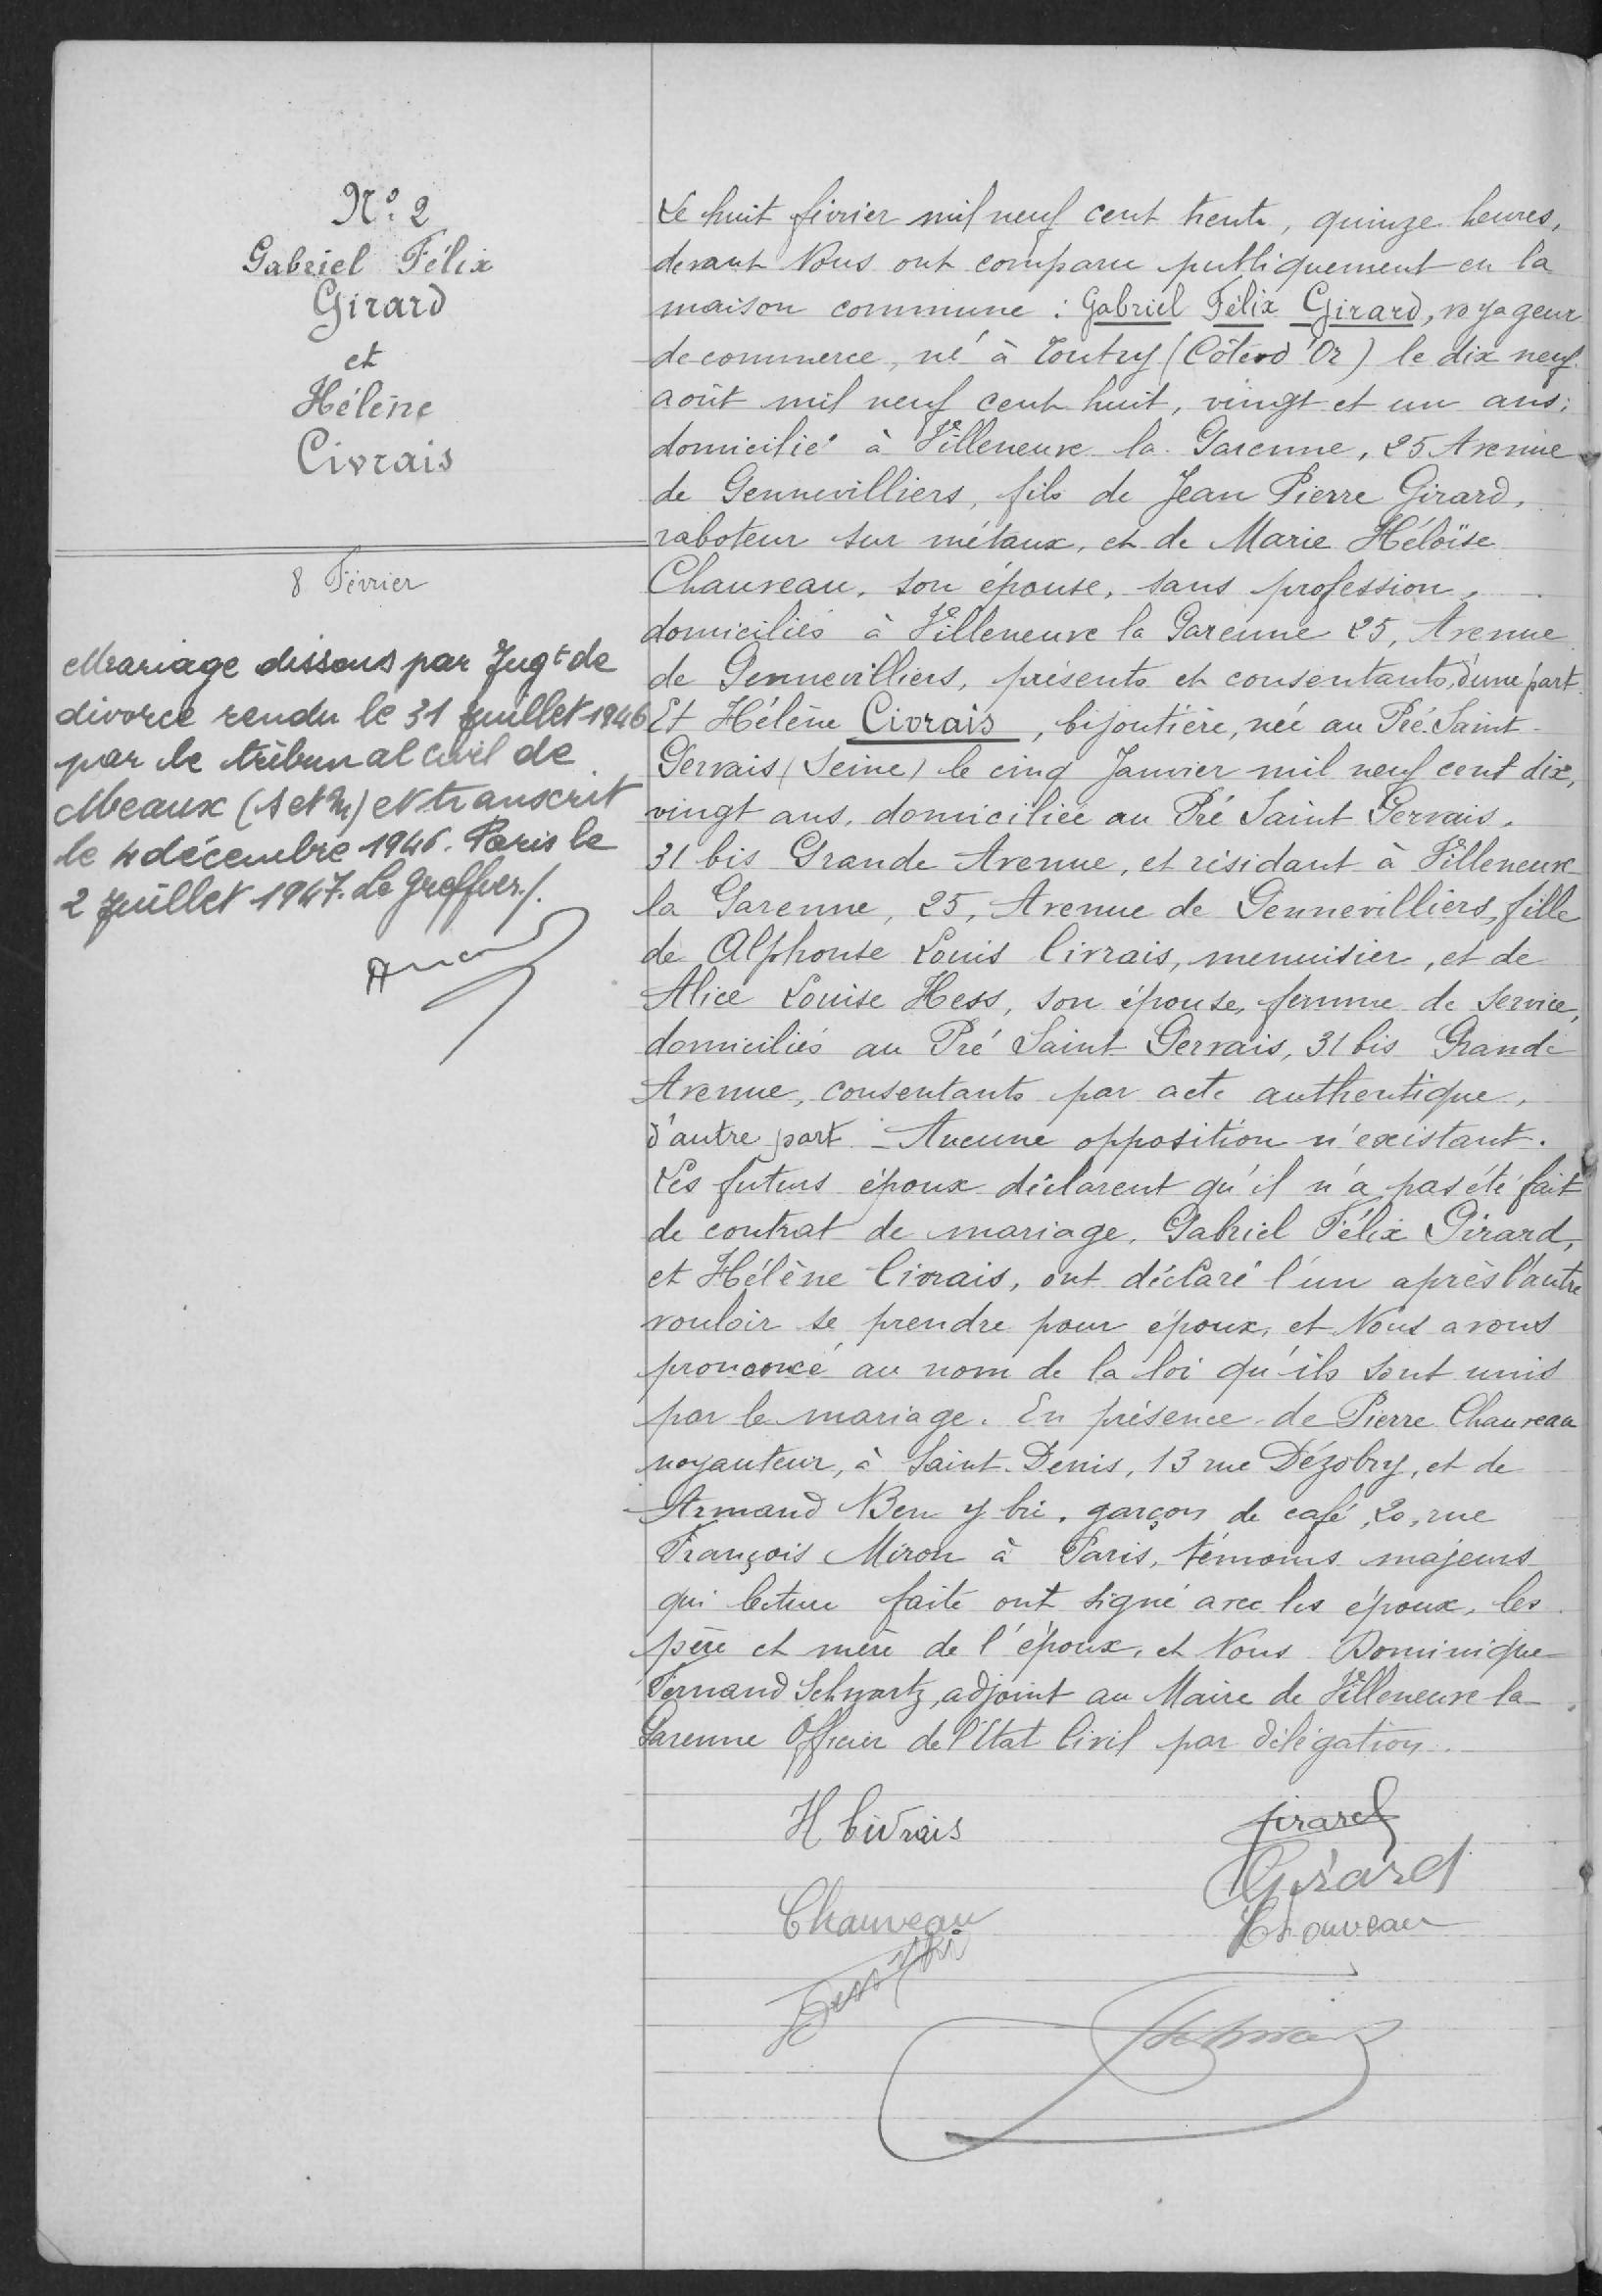N°2
Gabriel Félix
Girard
et
Hélène
Civrais
Le huit février mil neuf cent trente, quinze heures,
devant Nous ont comparu publiquement en la
maison commune: Gabriel Félix Girard, voyageur
de commerce, né à Toutry (Côte-d'Or) le dix neuf
août mil neuf cent huit, vingt et un ans;
domicilié à Villeneuve la Garenne, 25 Avenue
de Gennevilliers, fils de Jean Pierre Girard,
raboteur sur métaux, et de Marie Héloïse
Chauveau, son épouse, sans profession,
domiciliés à Villeuneuve la Garenne 25, Avenue
de Gennevilliers, présents et consentant, d'une part.
Et Hélène Civrais, bijoutières, née à Pré-Saint
Gévrais (Seine) le cinq Janvier mil neuf cent dix,
vingt ans, domiciliée au Pré Saint Gervais.
31 bis Grande Avenue, et résidant à Villeneuve
la Garenne, 25, Avenue de Gennevilliers, fille
de Alphonse Louis Civrais, menuisier, et de
Alice Louise Hess, son épousé femme de service
domiciliés au Pré Saint Gervais, 31 bis Grande
Avenue, consentants par acte authentique
d'autre part. Aucune opposition n'existant
Les futurs époux déclarent qu'il n'a pas été fait
de contrat de mariage. Gabriel Félix Gérard,
et Hélène Civrais, ont déclaré l'un après l'autre
vouloir se prendre pour époux et Nous avons
prononcé au nom de la loi qu'ils sont unis
par le mariage. En présence de Pierre Chaureau
noyauteur, à Saint-Denis, 13 rue Dézoby, et de
Armand Ben Y bi, garçon de café, 20, rue
François Miron à Paris, témoins majeurs
qui lecture faite ont signé avec les époux, les
père et mère de l'épouse et Nous Dominique
Fernand Schwartz, adjoint au maire Villeneuve la
Garenne Officier de l'Etat Civil par délégation
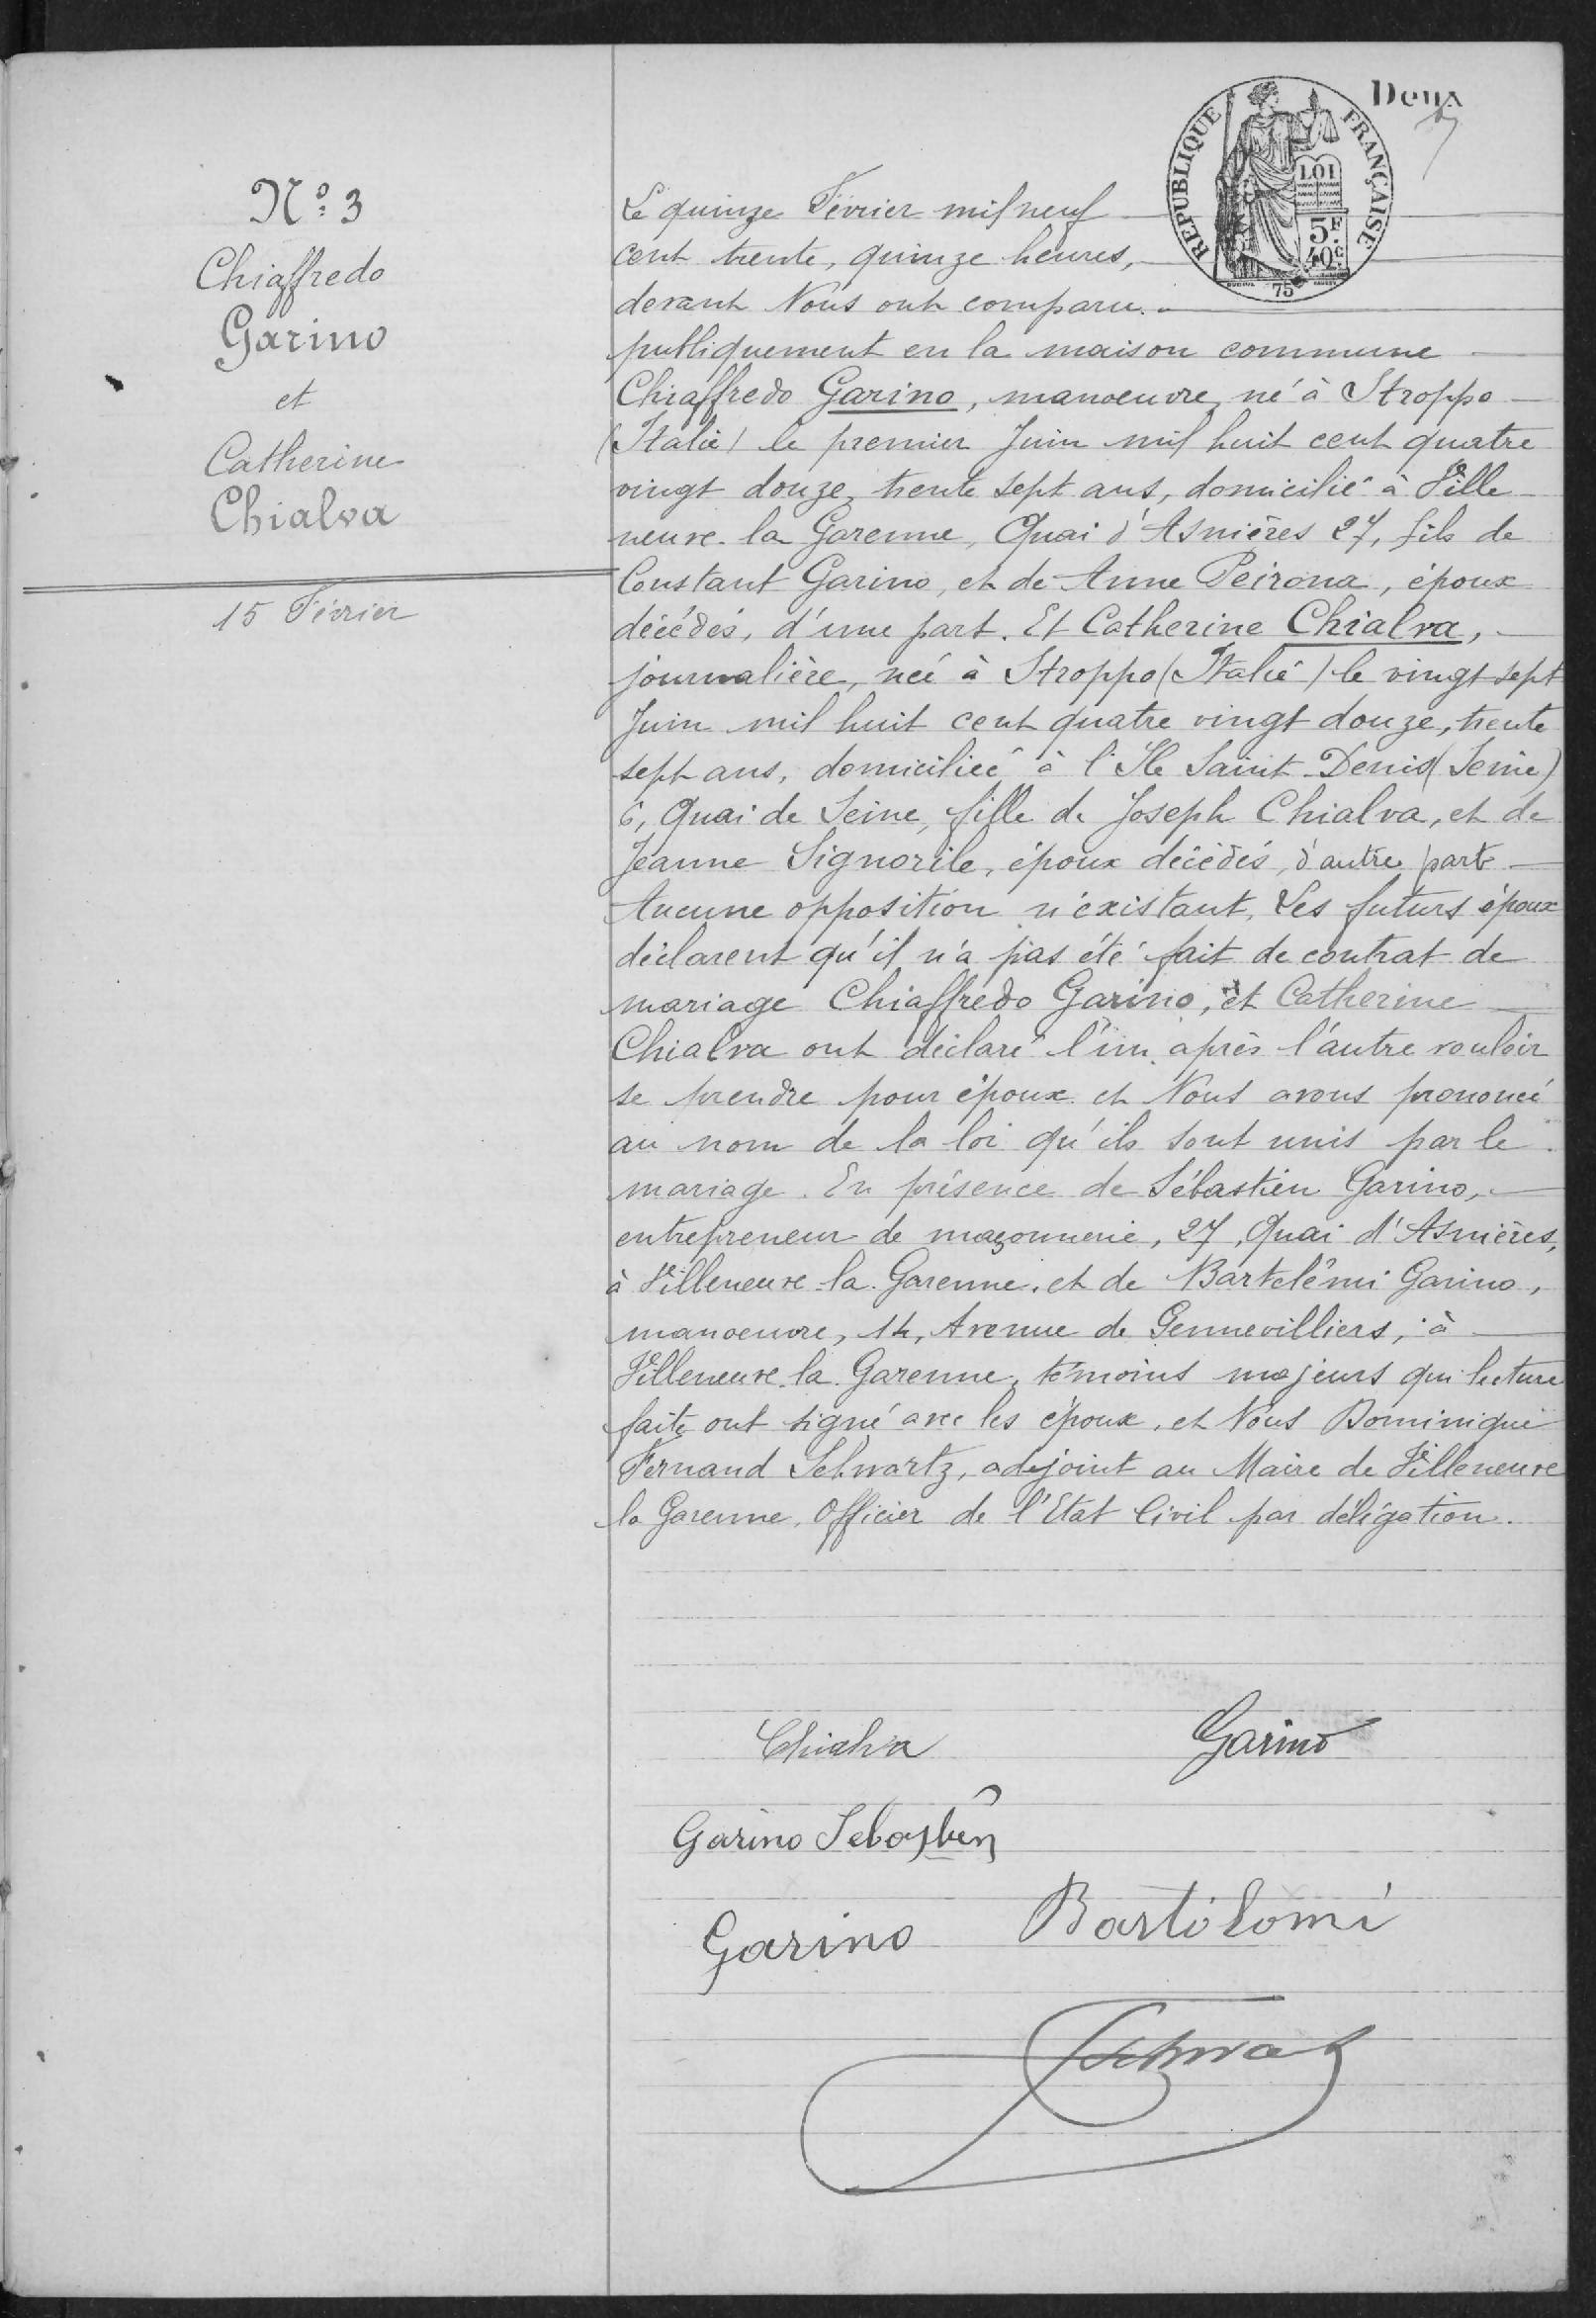N°3
Chiaffredo
Garino
et
Catherine
Chialva
15 février
Le quinze février mil neuf -
cent trente, quinze heures, -
devant Nous ont comparu -
publiquement en la maison commune -
Chiaffrdo Gorino, manoeuvre, né à Stroppo -
(Italie) le premier Juin mil huit cent quatre
vingt douze trente sept ans, domicilié à Ville
neuve la Garenne, Quai d'Asnières 27, fils de
Constant Garino, et de Anne Peirona, époux
décédés, d'une part. Et Catherine Chialva, -
journalière, née à Stroppo (Italie) le vingt-sept
Juin mil huit cent quatre vingt-douze, trente
sept ans, domiciliée à l'Ile Seint-Denis (Seine)
6, Quai de Seine, fille de Joseph Chialva, et de
Jeanne Signorile, époux décédés, d'autre part -
Aucune opposition n'existant, Les futurs époux
déclarent qu'il n'a pas été fait de contrat de
mariage Chiaffredo Garino, et Catherine -
Chialva ont déclaré l'un après l'autre vouloir
se prendre pour époux et Nous avons prononcé
au nom de la loi qu'ils sont unis par le
mariage. En présence de Sébastien Garino, -
entrepreneur de maçonnerie, 27, Quai d'Asnières,
à Villeneuve-la-Garenne, et de Bartelèmi Garino,
manoeuvre, 14, Avenue de Gennevilliers, à -
Villeneuve-la-Garenne, témoins majeurs qui lecture
faite ont signé avec les époux. et Nous Dominique
Fernand Schwartz, adjoint au Maire de Villeneuve
la Garenne. Officie de l'Etat Civil par délagation.